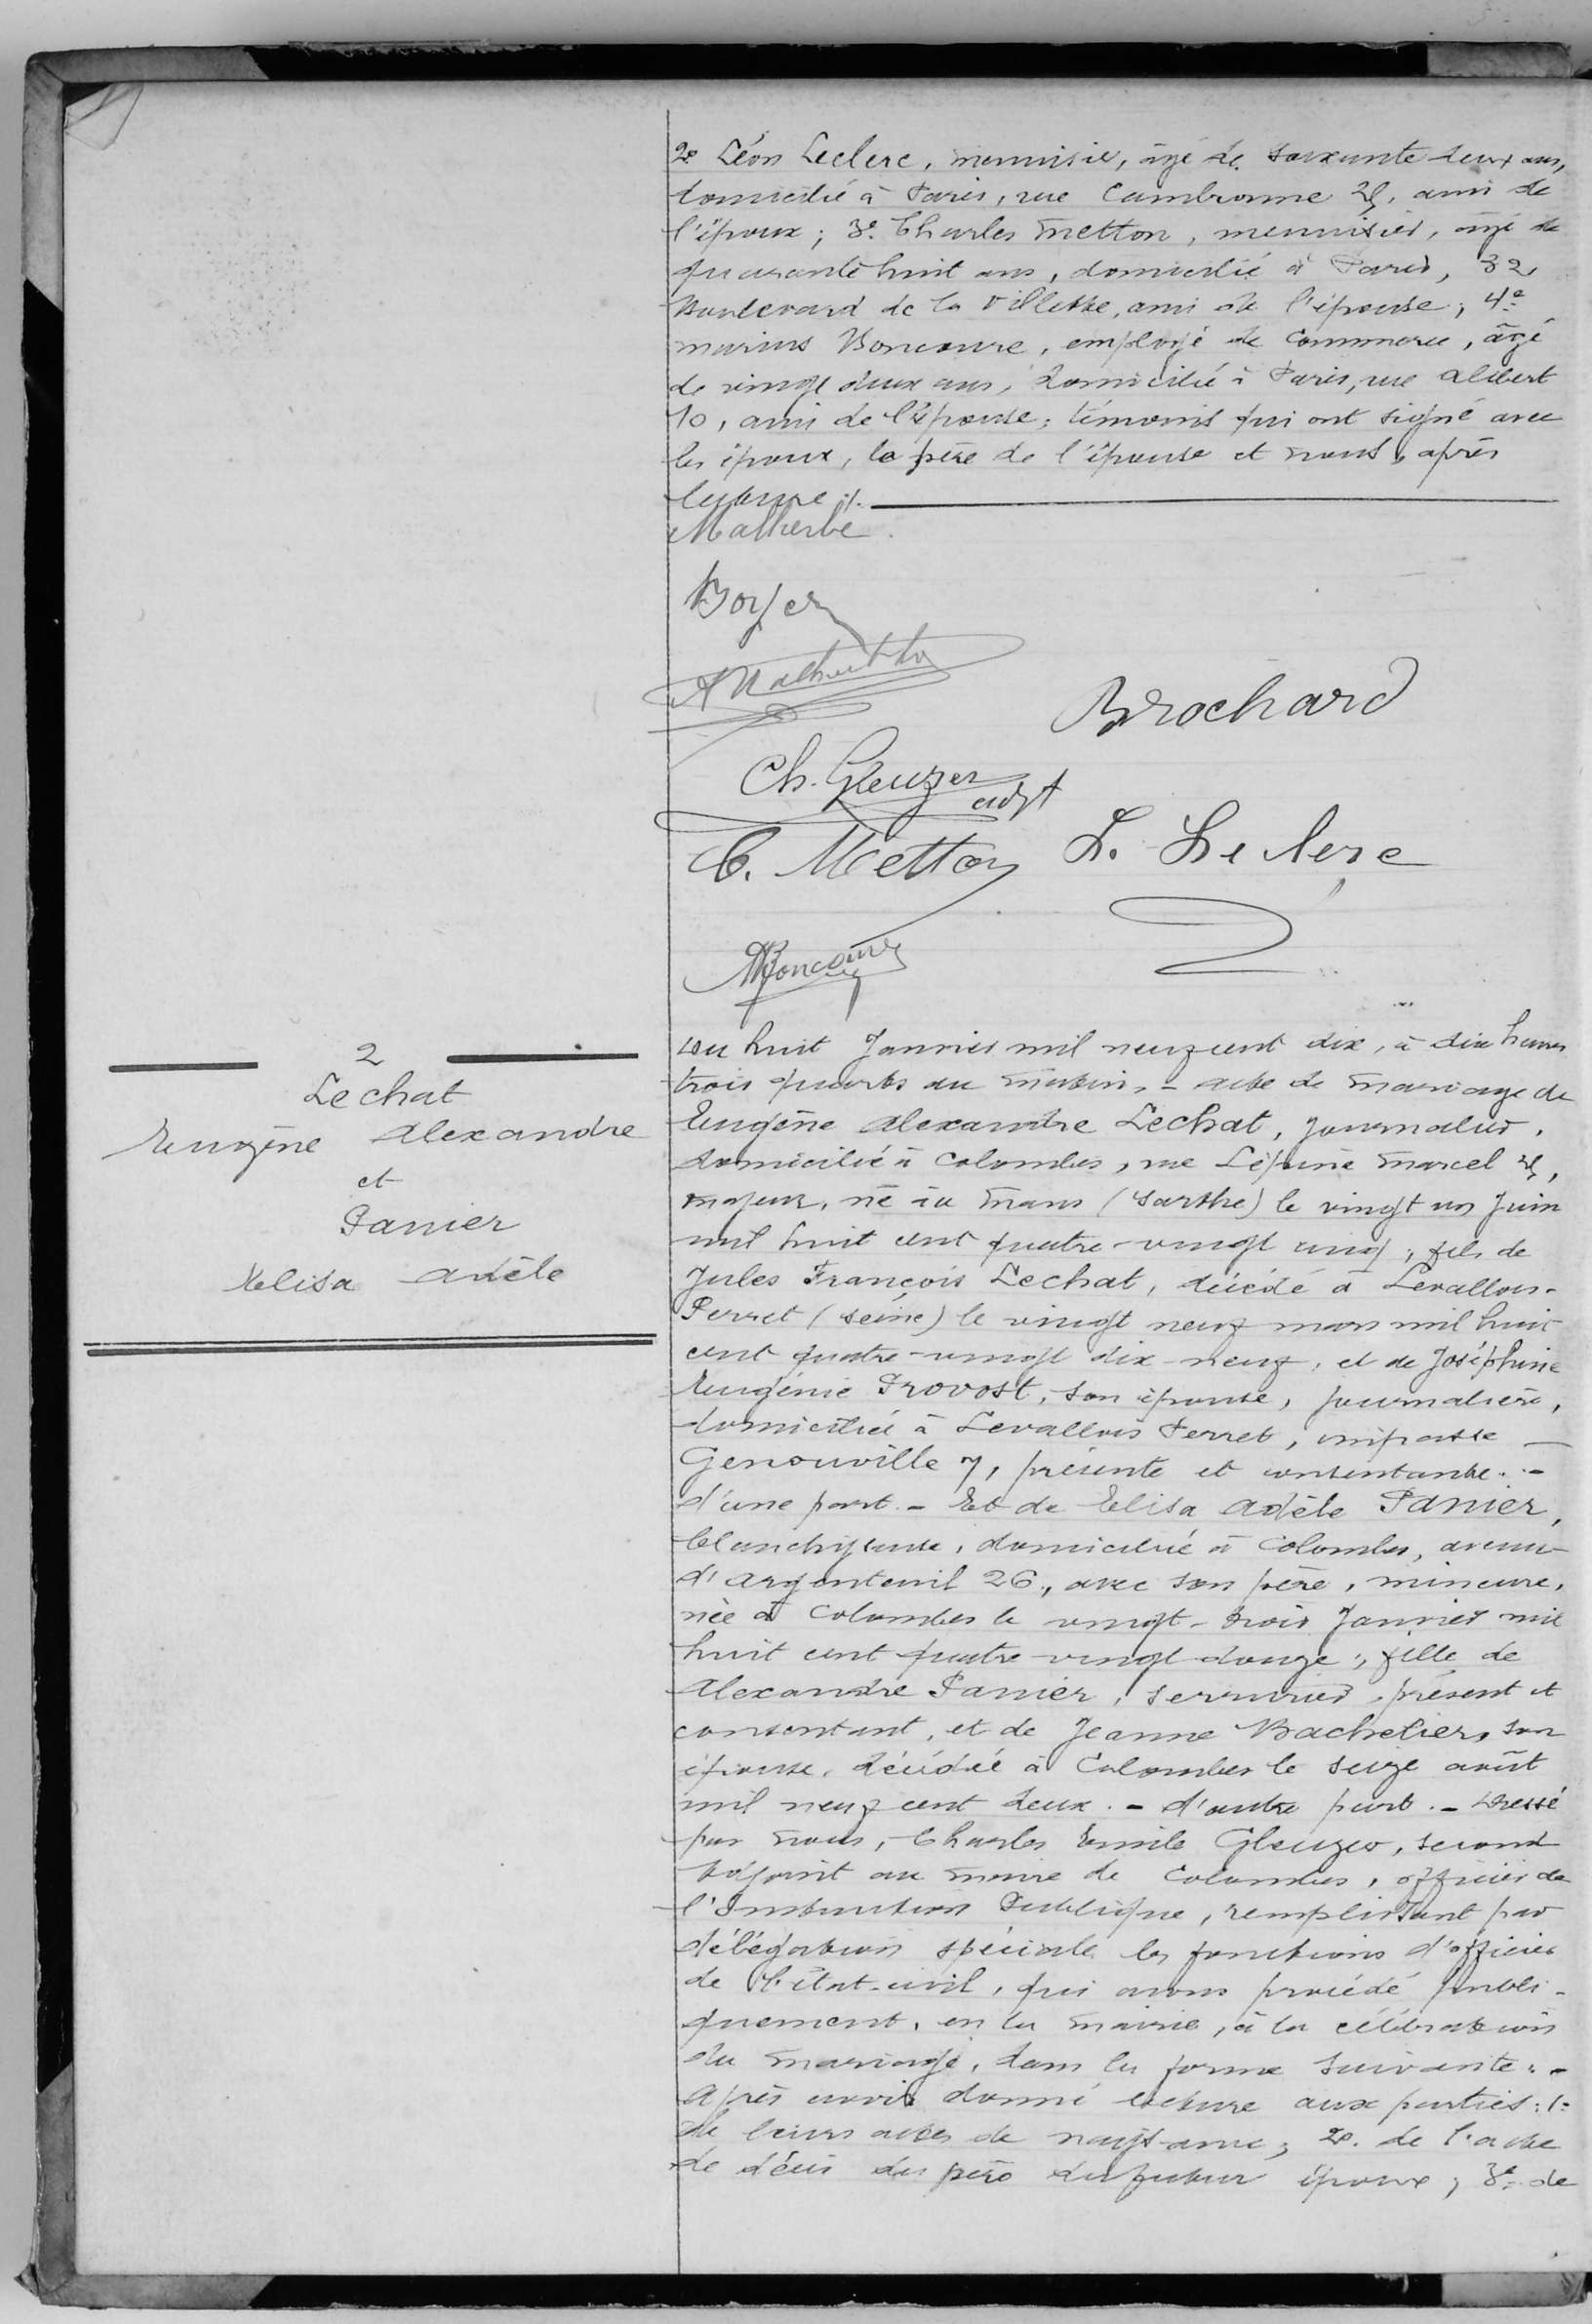2° Léon Leclerc, menuisier, âgé de soixante-deux ans,
domicilié à Paris, rue Cambronne 25, ami de
l'époux; 3° Charle Metton, menuisier, âgé de
quarante huit ans, domicilié à Paris, 32,
Boulevard de la Villette, ami de l'épouse; 4°
marius Bonconne, employé de commerce, âgé
de vingt deux ans, domicilié à Paris, rue Albert
10, ami de l'épouse; témoins qui ont signé avec
les époux, le père de l'épouse et nous, après
l'épouse ./.-
Malherbe
2
Le chat
Eugène Alexandre
et
Panier
Elisa Anèle
Le huit Janvier mil neuf cent dix, à dix heures
trois quarts du matin, acte de mariage de
Eugène Alexandre Lechat, journalier,
domicilié à Colombe, rue Lépine Marcel 25,
majeur, né au Mans (Sarthe) le vingt un Juin
mil heut cent quatre vingt cinq, fils de
Jules François Lechat, décédé à Levallois-
Perret (Seine) le vingt neuf mars mil huit
cent quatre-vingt dix-neuf, et de Joséphine
Eugénie Provost, son épouse, journalière,
domiciliée à Levallois Perret, impasse -
Genouville 7, présente et consentante
d'une part. Et de Elisa Adèle Panier,
blanchisseuse, domiciliée à Colombes, avenue
d'Argenteuil 26, avec son père, mineure,
née à Colombes le vingt-trois Janvier mil
huit cent quatre vingt douze, fille de
Alexandre Panier, serrurier, présent et
consentant, et de Jeanne Bachelier son
épouse, décédée à Colombes le seize août
mil neuf cent deux. d'autre part. Dressé
par nous, Charles Basile Gleuzeo, second
adjoint au maire de Colombes, officier de
l'Instruction publique, remplissant par
délégation spéciale les fonctions d'officier
de l'état civil, nous avons procédé publi
quement, en la mairie, à la célébration
du mariage, dans la forme suivante:
après avoir donné lecture aux parties: 1°
de leurs actes de naissance, 2° de l'acte
de décès du père du futur époux, 3° de
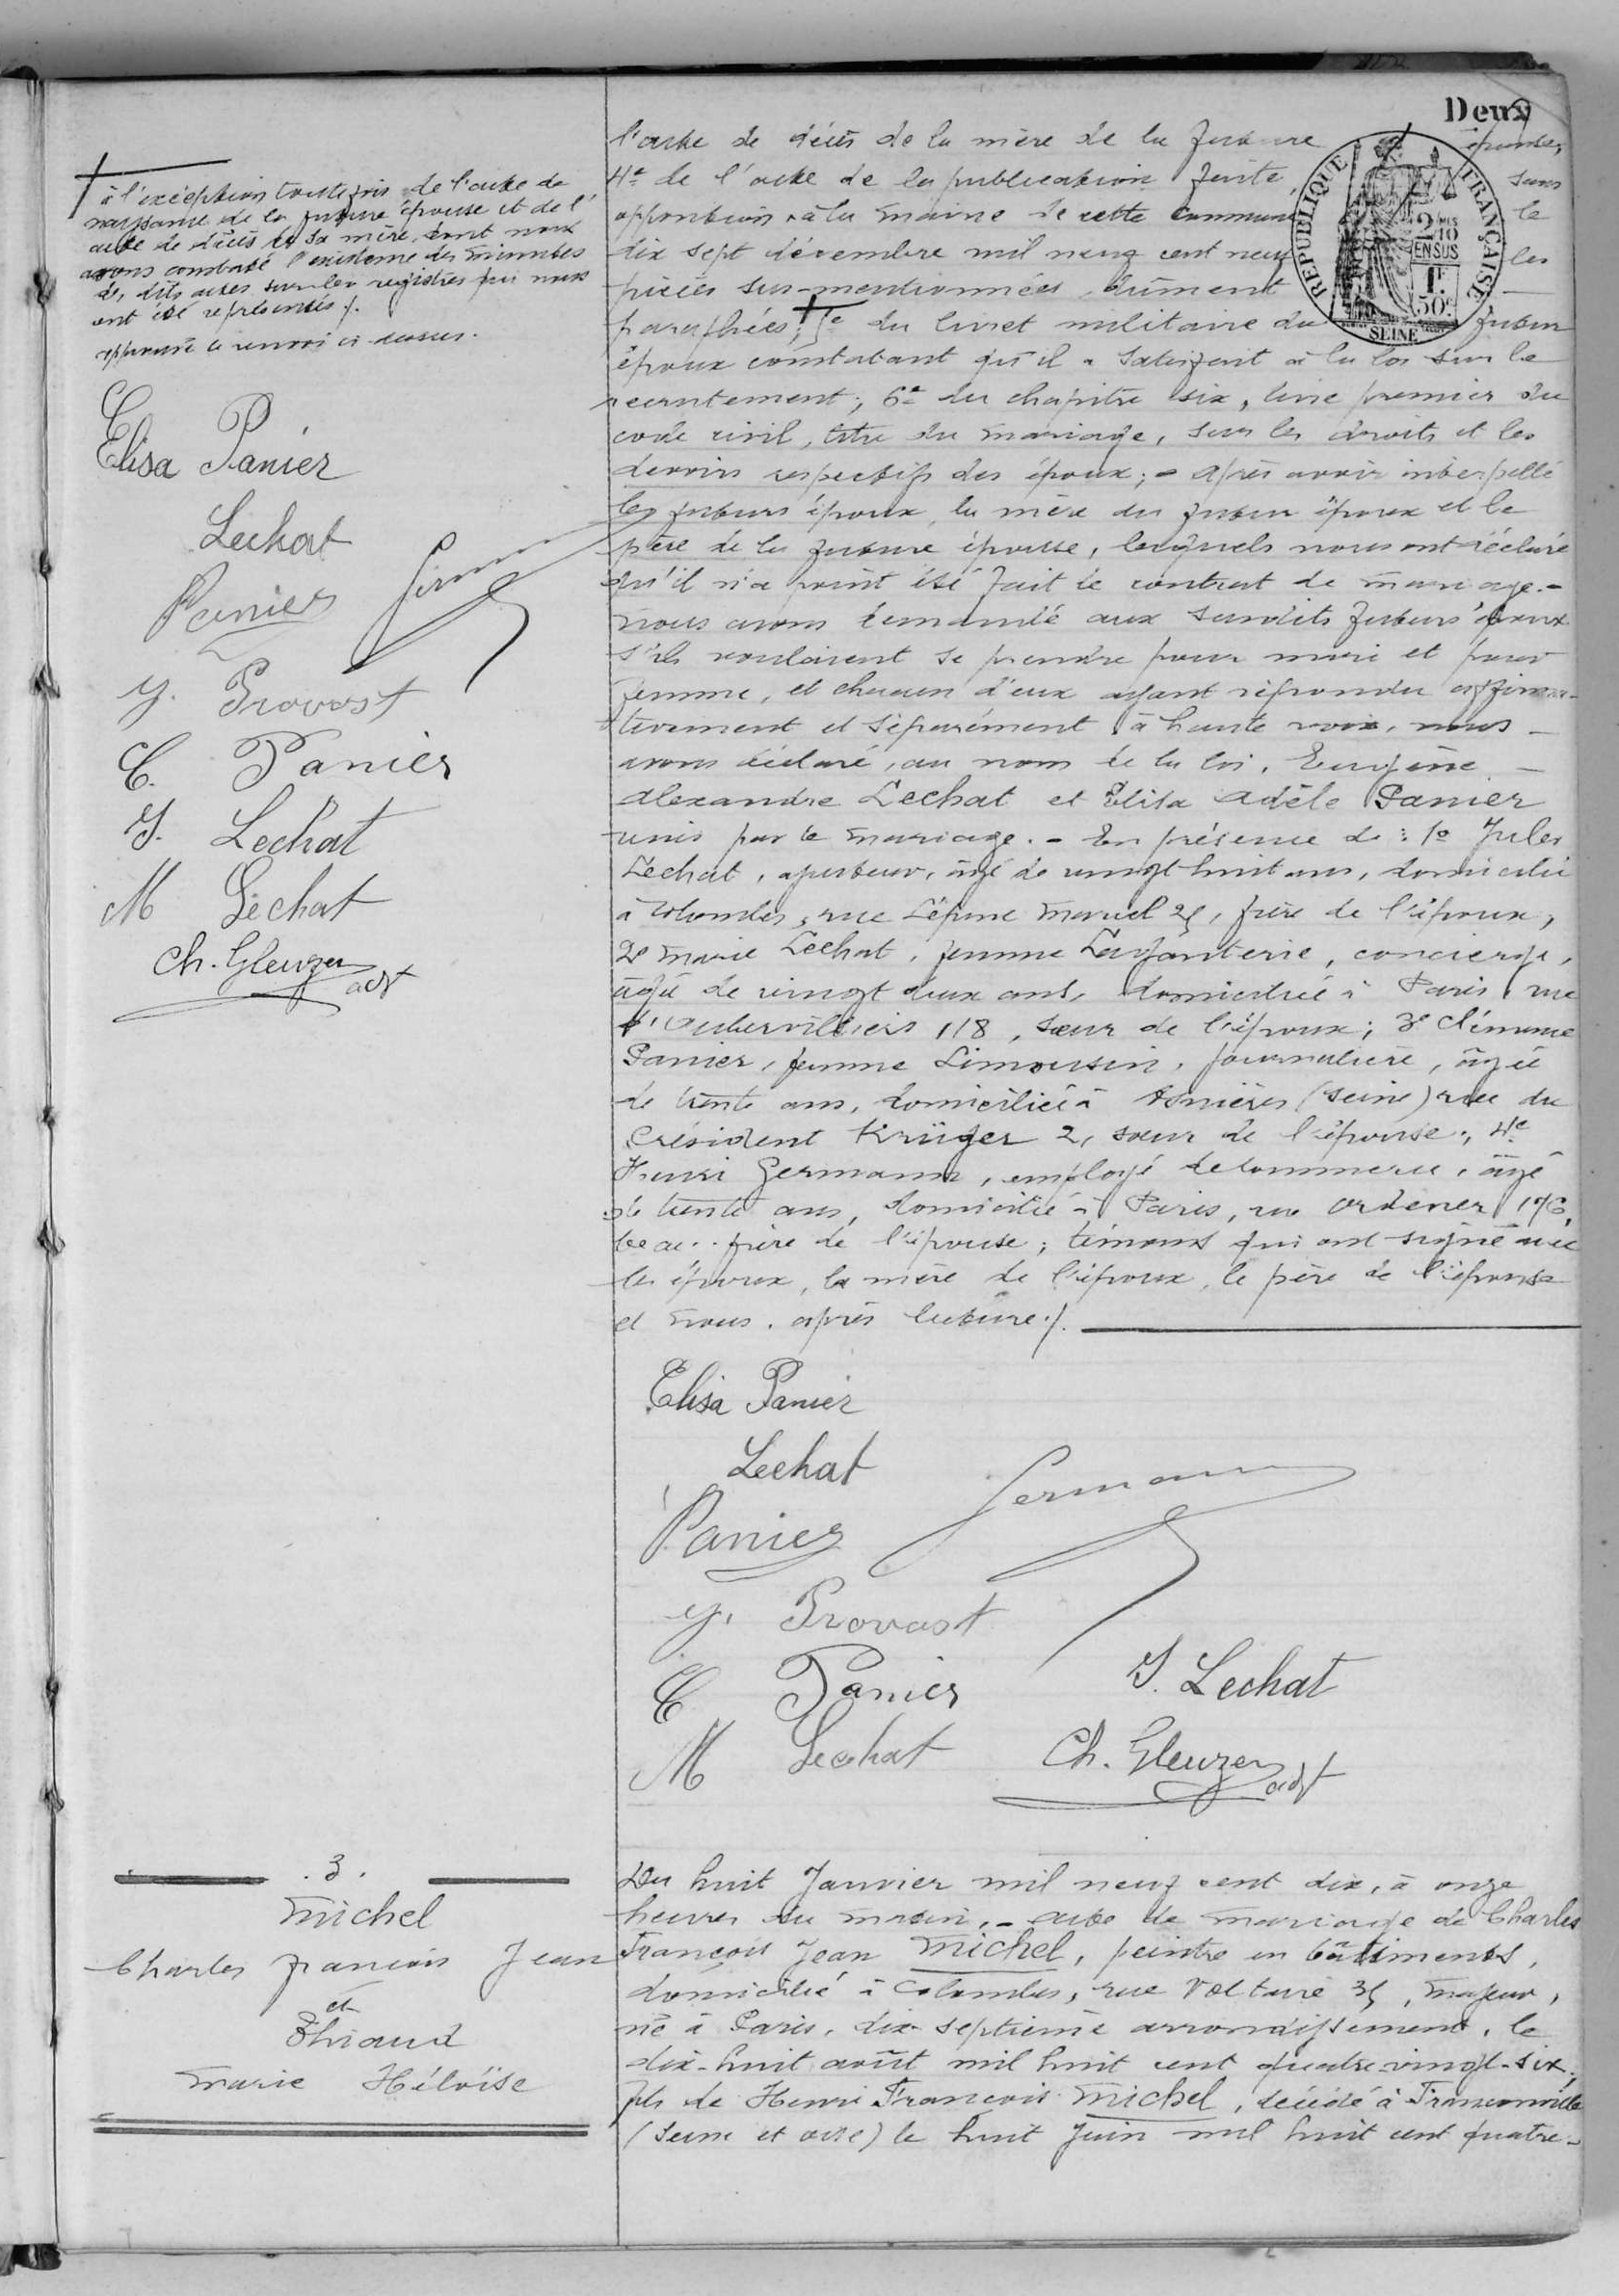-
l'acte de décès de la mère de la future épouse,
4° de l'acte de la publication faite sans
affirmation à la mairie de cette Commune le
dix sept décembre mil cinq cent neuf les
pièces sus-mentionnées dûment-
paraphées; 5° du livret militaire du futur
époux constatant qu'il a satisfait à la loi sur le
recrutement; 6° du chapitre six, livre premier du
code civil, titre du mariage, sur les droits et les
devoirs respectifs des époux; après avoir interpellé
les futurs époux, la mère du futur époux et le
père de la future épouse, lesquels nous ont déclaré
qu'il n'a point été fait de contrat de mariage.-
nous avons demandé aux susdits futurs époux
s'ils voulaient se prendre pour mari et pour
femme, et chacun d'eux ayant répondu affirma-
tivement et séparément à haute voix, nous-
avons déclaré, au nom de la loi, Eugène-
Alexandre Lechat et Elisa Adèle Panier
unis par le mariage.-En présence de: 1° Jules
Lechat, ajusteur, âgé de vingt huit ans, domicilié
à Colombes, rue Lépine Marcel 25, frère de l'épouse,
2° Marie Lechat, femme Laganterie, concierge,
âgé de vingt-deux ans, domicilié à Paris, rue
d'aubervilliers 118, soeur de l'épouse; 3° Clemence
Perrier, femme Limousin, journalière, âgée
de trente ans, domicilié à Asnières (Seine) rue du
président Krüger 2, soeur de l'épouse; 4°
Henri Germain, employé de commerce, âgé
de trente ans, domicilié à Paris, rue Ordener 176,
beau frère de l'épouse; témoins qui ont signé avec
les époux, la mère de l'époux, le père de l'épouse
et nous après lecture./.-
3
Michel
Charles François Jean
et
Thiand
Marie Héloise
Un huit Janvier mil neuf cent dix, à onze
heures du matin-acte de mariage de Charles
François Jean Michel, peintre en bâtiments,
domicilié à Colombes, rue Voltaire 36, majeur
né à Paris, dix septième arrondissement, le
dix-huit août mil huit cent quatre-vingt-six
fils de Henri François Michel, décédé à Franconville
(Seine et Oise) le huit juin mil huit cent quatre-